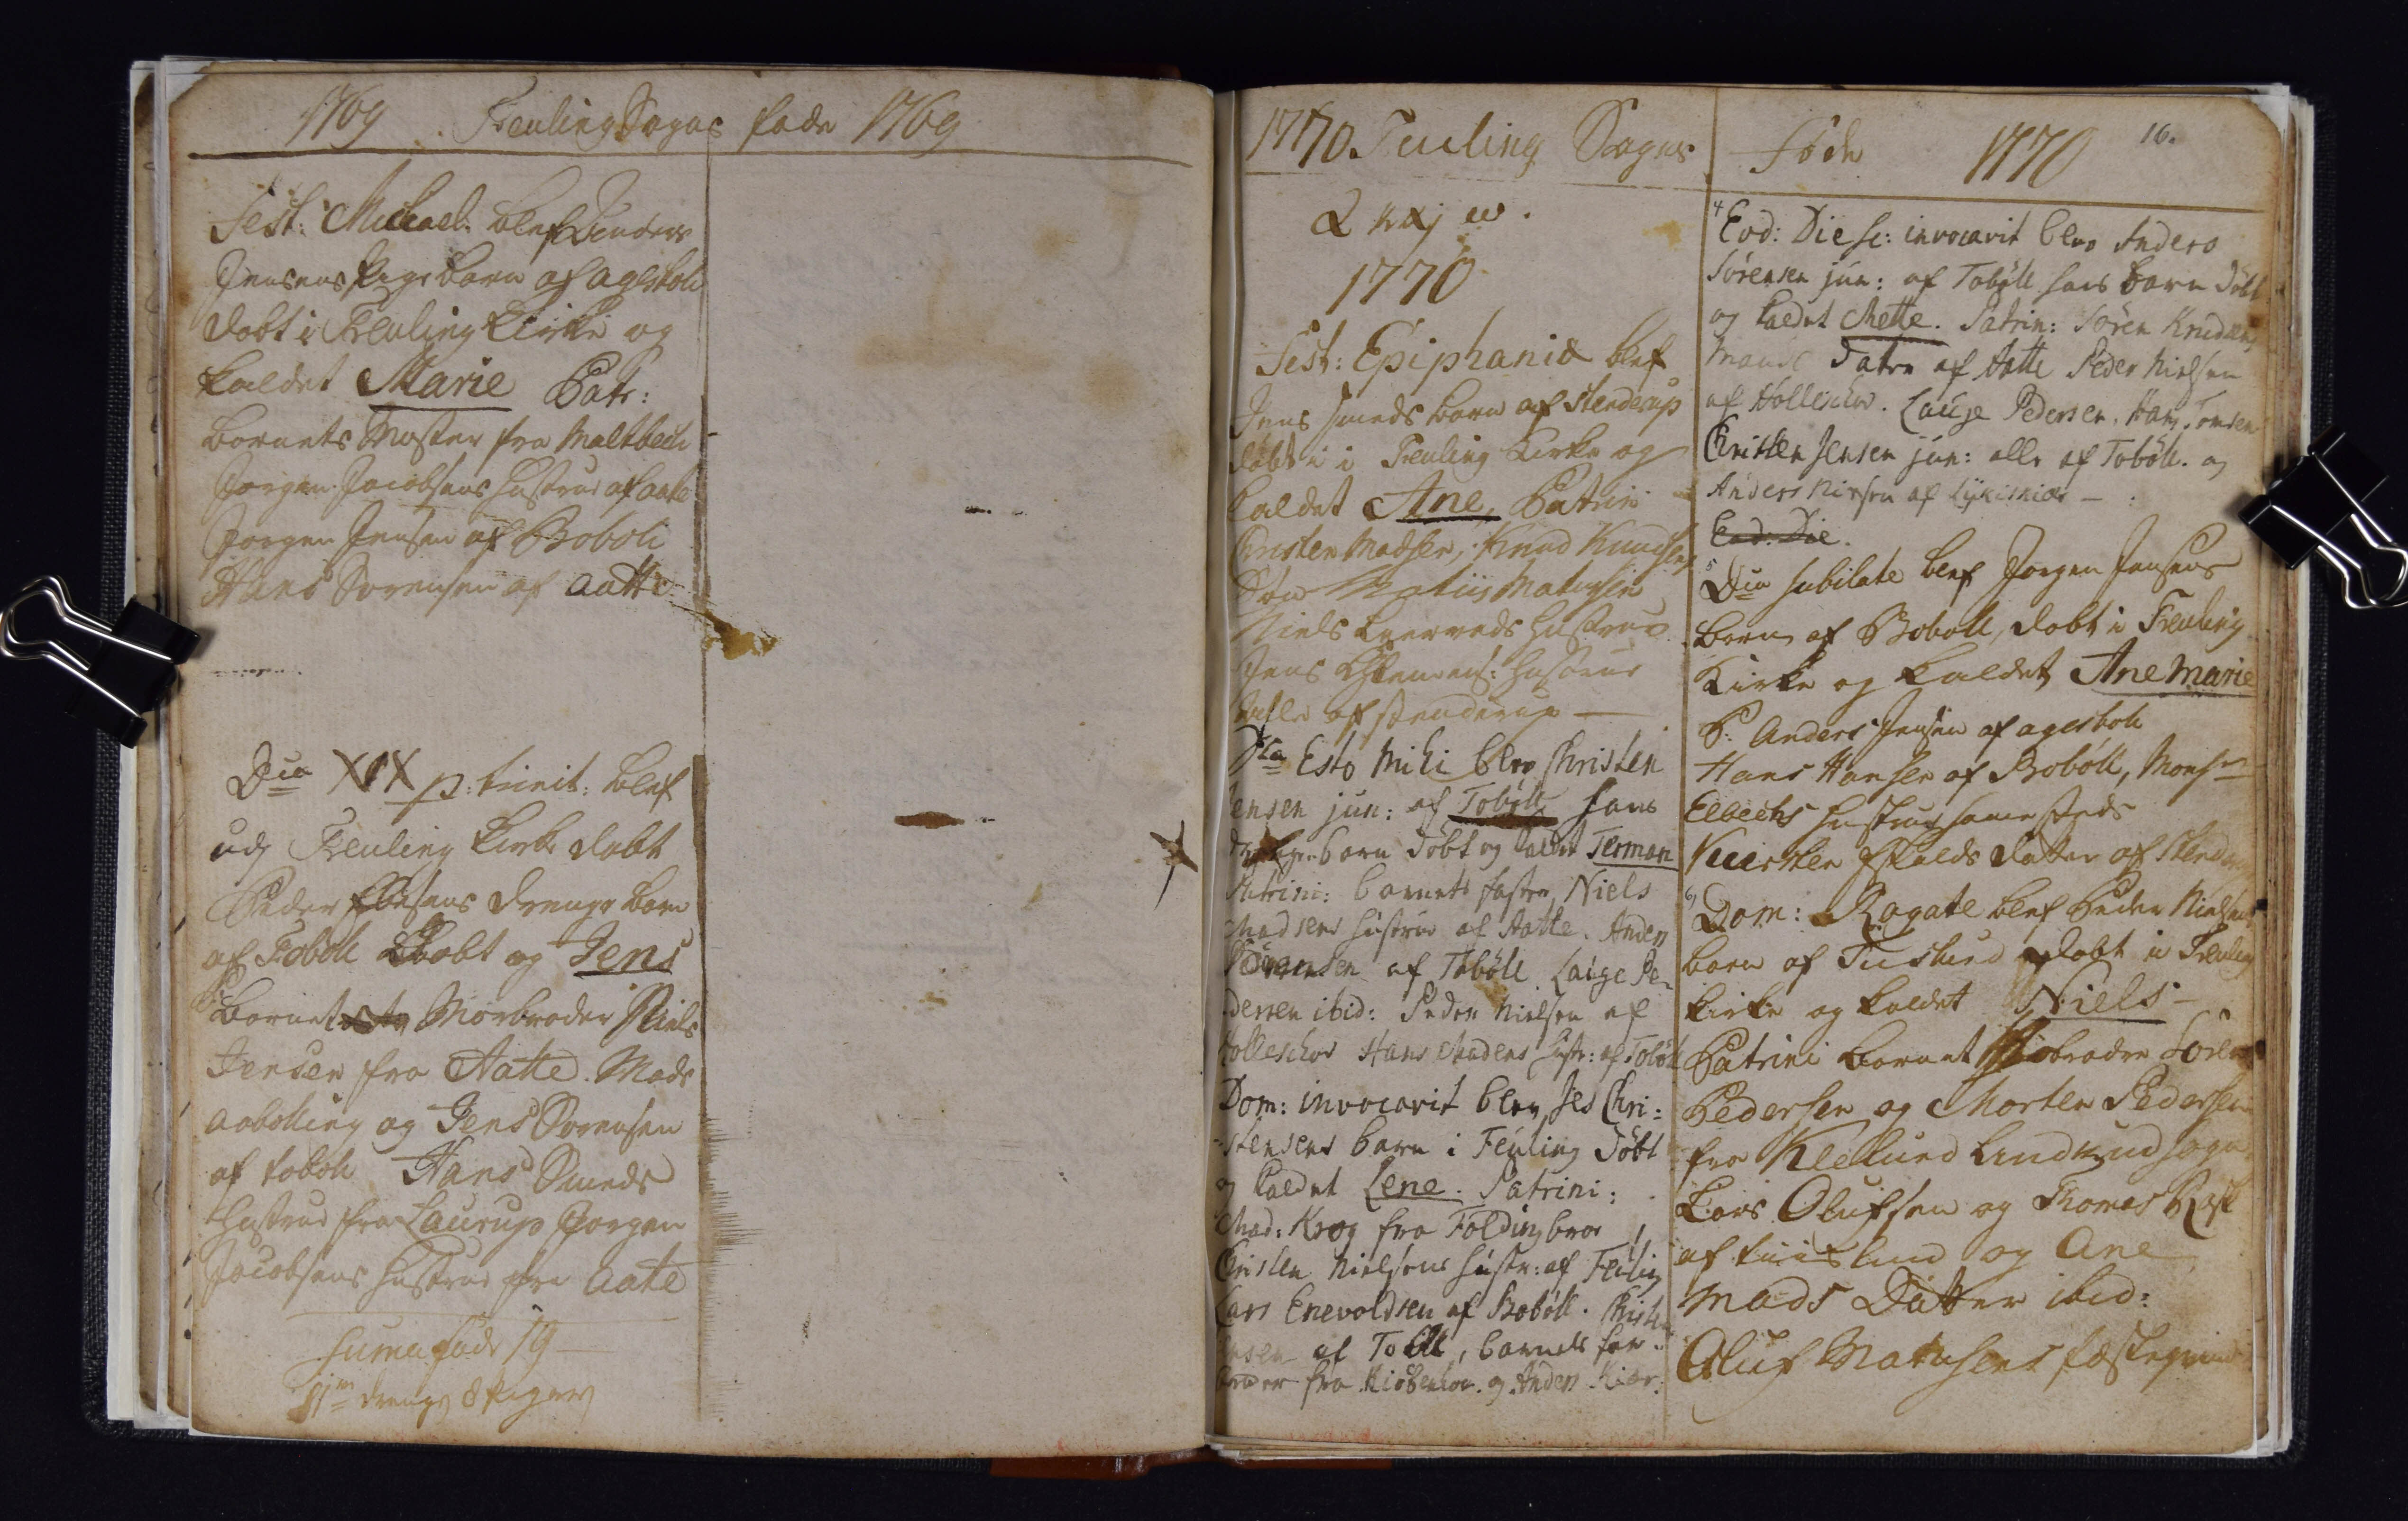1769
Feuling Sogns fode 1769
Fest. Michael blef Anders
Jensens Pige Barn af Agesboll
døbt i Feuling Kirke og
kaldet Marie Patr:
Bornets Moster fra Matbech
Jørgen Jacobsens Hustrue af Aatte
Jørgen Jensen af Boboøll
Hans Sørensen af Aatte
Dca XIX p: Trinit: Blev
udj Feuling Kirke dobt
Peder Ebesens Drenge Barn
af Toboll Dobt og Jens
Bornets ## Morbroder, Niels
Jensen fra Aatte. Mads
Aabolling og Jens Sørensen
af Tobol, Hans Smeds
Hustrue fra Laurup Jorgen
Jacobsens Hustrue fra Aatte
Summa fød 19
11## Drenge 8 Piger
1770 Feuiling Sogn.
## ## ##.
1770
Fest: Epiphaniæ blef
Jens Smeds Barn af Stenderup
døbt i i Feuling Kirke og
kaldet Ane Patrini
Christen Madsen, Knud Knudsens
Søn Matiis Matiasen
Niels Leervads Hustrue
Jens Klemens: Hustrue
Alle af stenderup
Dca Esto Mihi blev Christen
Jensen Jun: af Tobøll hans
drengebarn døbt og kaldet Terman
Patrini: barnet faster, Niels
Madsens Hustrue af Aatte. Ander
Sørensen af Tobøll, Lauge Pe¬
dersen ibid: Peder Nielsen af
Holleschov Hans Madsens Hstr: af Tobøll
Dom: Invocavit blev Jes Chri¬
stensens barn i Feuling døbt
og kaldet Lene. Patrini:
Mad: Krog fra Folding broe
Christen Nielsens Hustr: af Feuling
Lars Enevoldsen af Bobøll. Christen
##sen af Tobøll, barnets far¬
broder fra Kiøbenhov. og Ander Kiær
16.
Føde
1770
4
Eod: Dies: invocavit blev Anders
Sørensen jun: af Tobøll hans barn døbt
og kaldet Mette. Patrin: Søren Knud##
Man## dater af Aatte Peder Nielsen
af Holleschov. Lauge Pedersen, Hans Tomsen
Christen Jensen jun: alle af Tobøll. og
Anders Nielsen af Lykiskiær -
Eod. Die.
5
Dca Jubilate blev Jorgen Jensens
Barn af Boboll, døbt i Feuling
Kirke og kaldet Ane Marie
P: Anders Jensøn af Agesboll
Hans Hansen af Bobøll, Monsr
Elbechs Hustrue sammesteds
Kiersten Eskilds Datter af Stenderup
6
Dom: Rogate blef Peder Nielsens
Barn af Tiislund døbt i Feuling
Kirke og Kaldet Niels.
Patrini Barnet Farbrodre Søren
Pedersen og Morten Pedersen
fra Klelund Lindknud Sogn
Lars Olufsen og Thomas Rafn
af Tiislund og Ane
Mads Datter ibid:
Oluf Mathisens Fæsteqvinde
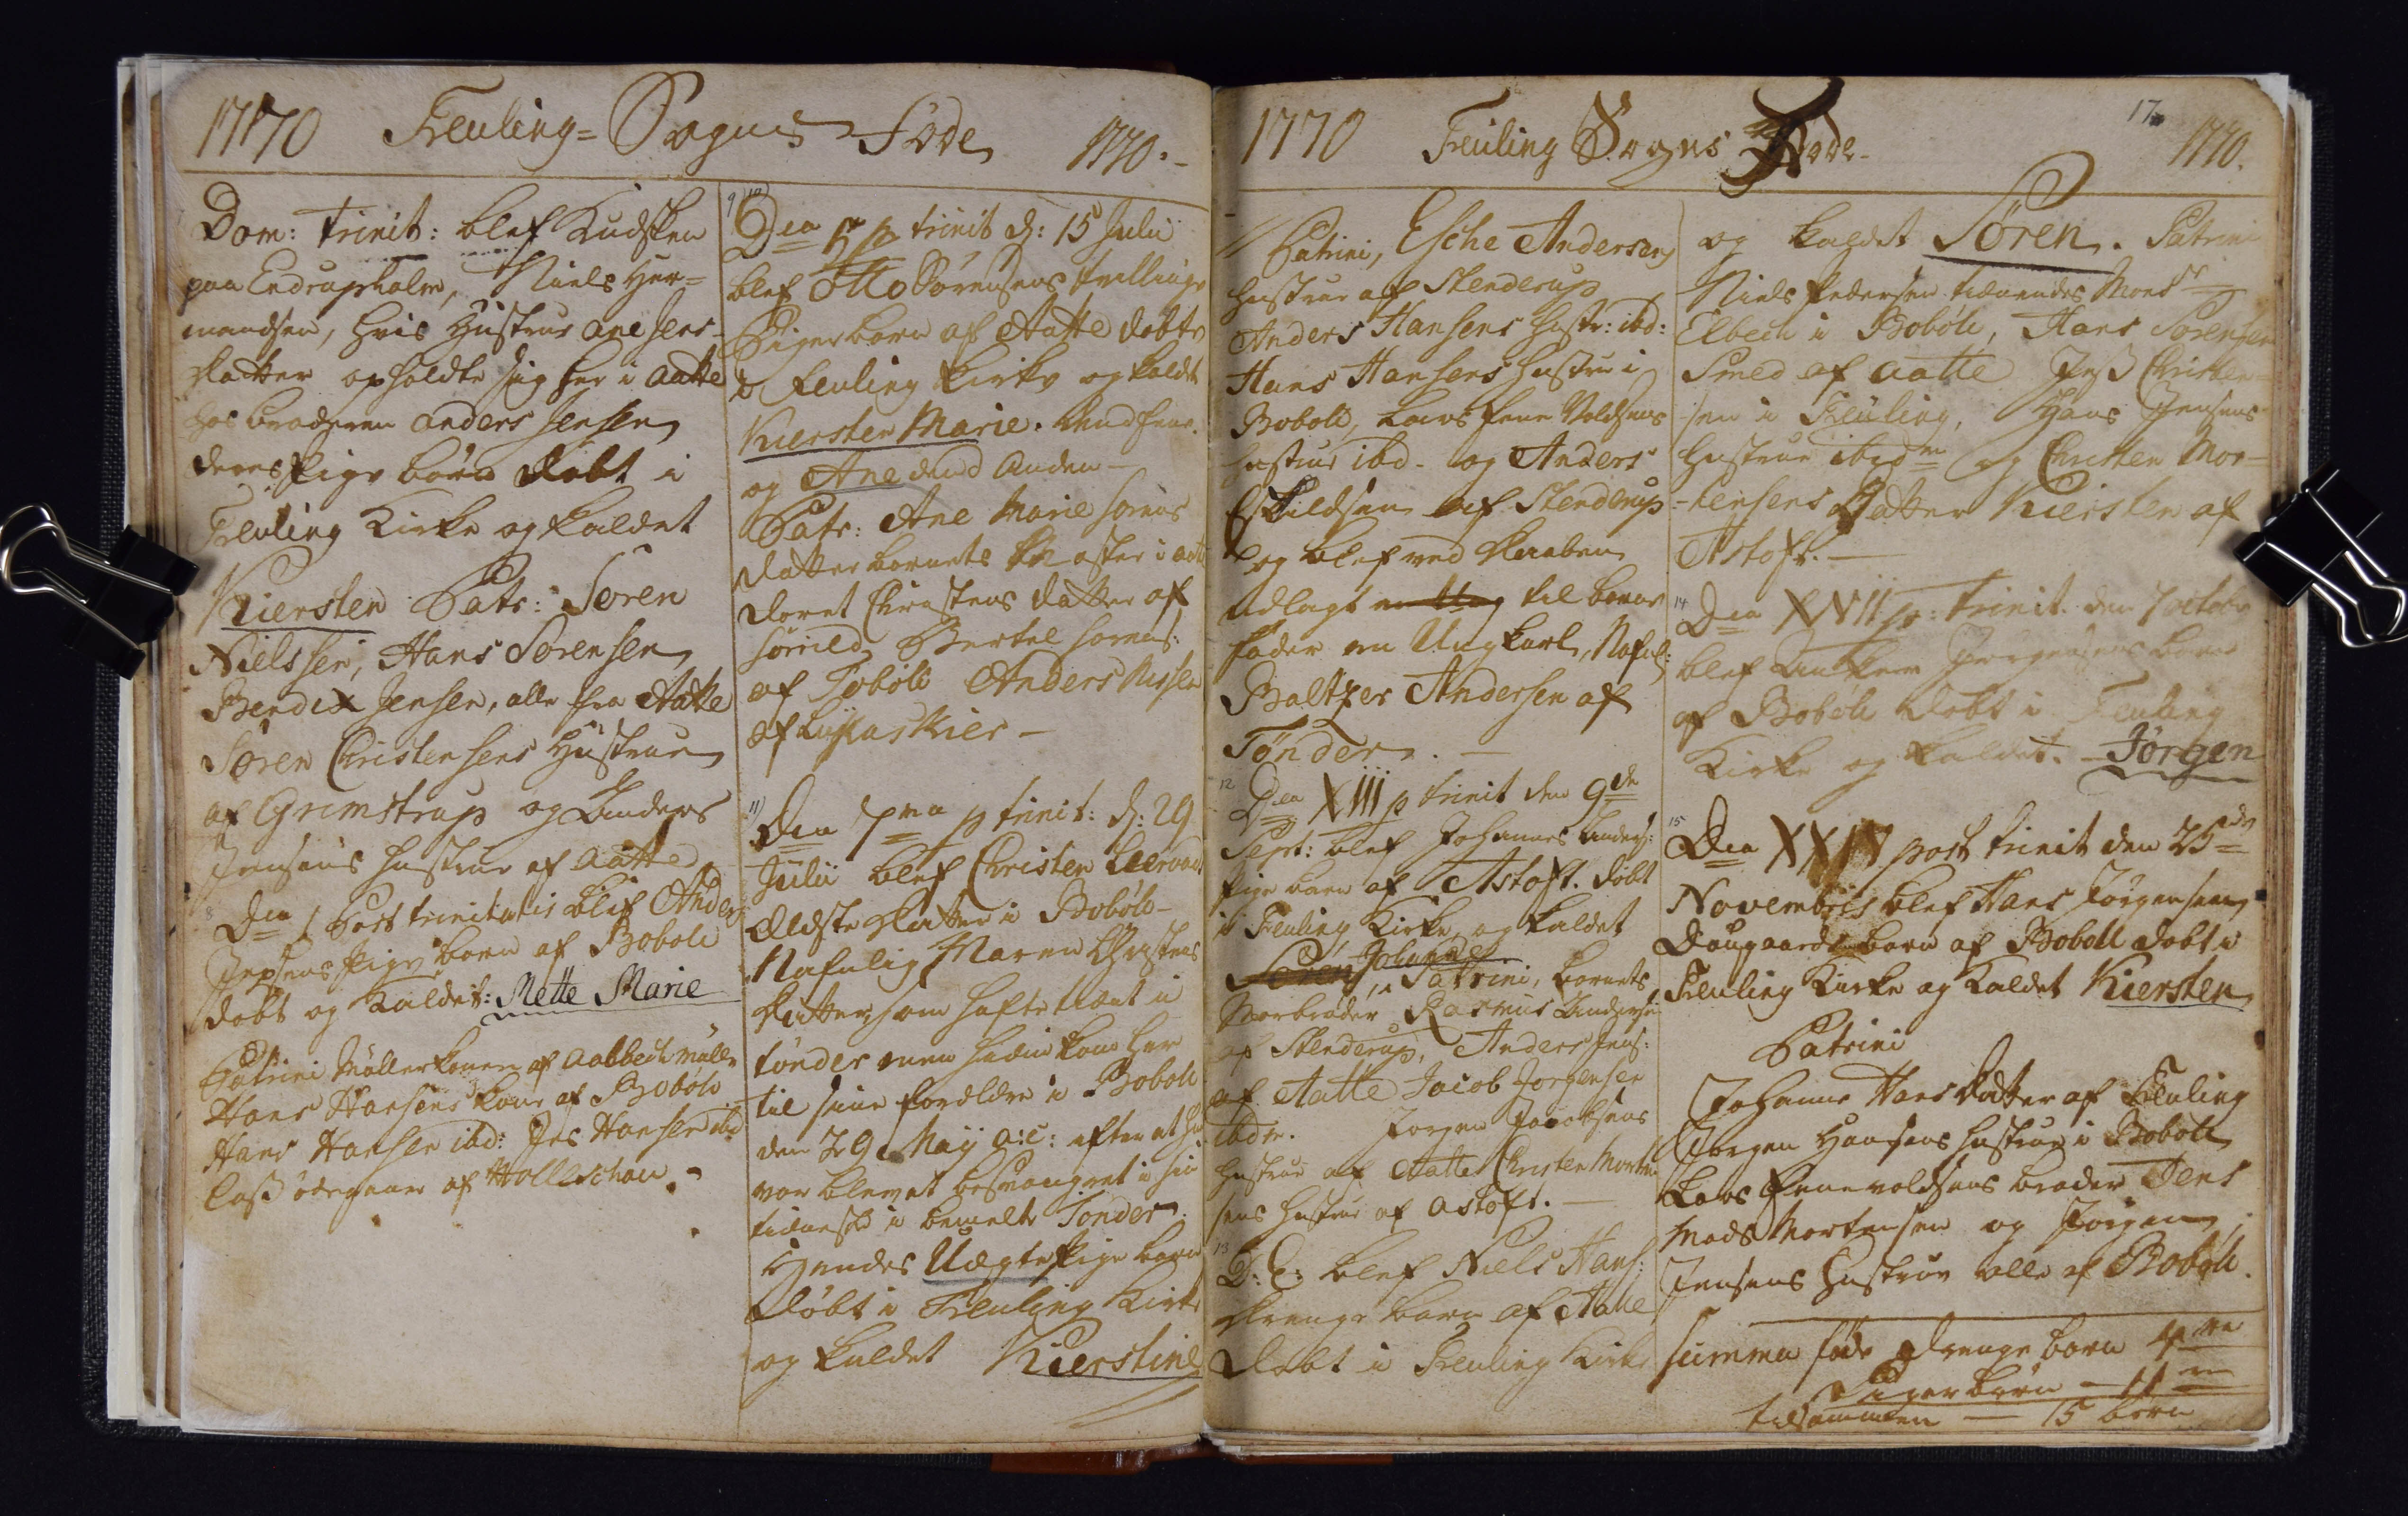1770 Feuling Sogns Føde
Dom: Trinit: blef Kudsken
paa Endrupholm, Niels Her¬
mandsen, hvis Hustrue Ane Jens¬
datter opholdte sig her i Aatte
hos Broderen Anders Jensen
deres Pige Barn døbt i
Feuling Kirke og kaldet
Kiersten Patr: Soren
Nielsen, Hans Sorensen
Bendix Jensen, alle fra Aatte
Søren Christensens Hustrue
af Grimstrup og Anders
Jensens Hustrue af Aatte
8
Dca 1 Post Trinitatis blef Anders
Jepsens Pige Barn af Boboll
døbt og kaldet: Mette Marie
Patrini Møllerkonen af Aabeth Mølle
Hans Hansens Kone af Bobøll
Hans Hansen ibd: Jes Hansen ibd
Lass ødegaar af Holleschou
1770 
10)
Dca 5 p Trinit: d: 15 Julii
blef Otto Sørensens tvillinge
Pige Born af Aatte døbte
i Feuling Kirke og kaldet
Kiersten Marie. dend Eene
og Ane dend Anden
Patr: Ane Marie Sorens
Datter Barnets ##ster i Aatte
Doret Christens Datter af
Sorrild, Bertel sorens:
af Tobøll Anders Nissen
af Lykaskier
11)
Dca 7ma p Trinit: d: 29
Julii blef Christen Leervads
Ældste Datter i Bobøll -
Nafnlig Maren Christens
Datter, som hafte tiænt i
Tønder men hade kom her
til sine forældre i Boboll
den 29 Maij A:e: efter at Hu 
var blevet besvangret i si##
ti## i bemelte Tonder
Hendes Uægte Pige Barn
døbt i Feuling Kirke
og kaldet Kierstine
1770 Feuling Sogns Føde
// Patrini, Esche Andersens
Hustrue af Stenderup
Anders Hansens Hustr: ibd:
Hans Hansens Hustru i
Bobold, Lars Eene Voldsens
Hustrue ibd. og Anders
Eskildsen af Stenderup
og blef ved Daaben
udlagt ## til barne¬
fader en Ungkarl, Nafnl:
Baltzer Andersen af
Tønder.
12.
Dca XIII p Trinit den 9de
Sept: blef Johannes Anders:
Pige Barn af Astoft. døbt
i Feuling Kirke, og kaldet
6
Johanne
Søren, Patrini, Bornets
Morbroder, Rasmus Andersens
af Stenderup, Anders Jens:
af Aatte Jacob Jorgensen
ibd. Jorgen Jacobsens
Hustrue af Aatte Christen Morten¬
sens Hustrue af Astoft.
13
D: E: blef Niels Hans:
Drenge Barn af Aatte
døbt i Feuling Kirke
17
1770
og kaldet Søren. Patrini
Niels Pedersen tiænendes Momsr
Elbech i Bobøll, Hans Sorensen
Smed af Aatte Jes Christen¬
sen i Feuling, Hans Jensens
Hustrue ibidm og Christen Mor¬
tensens Datter Kiersten af
Astoft.
14
Dca XVII p: Trinit. den 7 octobr
blef Anker Jorgensens Barn
af Bebøll døbt i Feuling
Kirke og kaldet. -  Jørgen
15
Dca XXIV post Trinit den 25de
Novembris blef Hans Jørgensens
Daugaards Barn af Boboll døbt i
Feuling Kirke og kaldet Kiersten
Patrini
Johanne Hans Datter af Feuling
Jørgen Hansens Hustrue i Boboll
Lars Eenevoldsens Broder Jens
Mads Mortensen og Jørgen
Jensens Hustrur alle af Bobøll
Summa føde Drenge Born 4ma
Piger Børn - 11##
tilsammen - 15 Børn